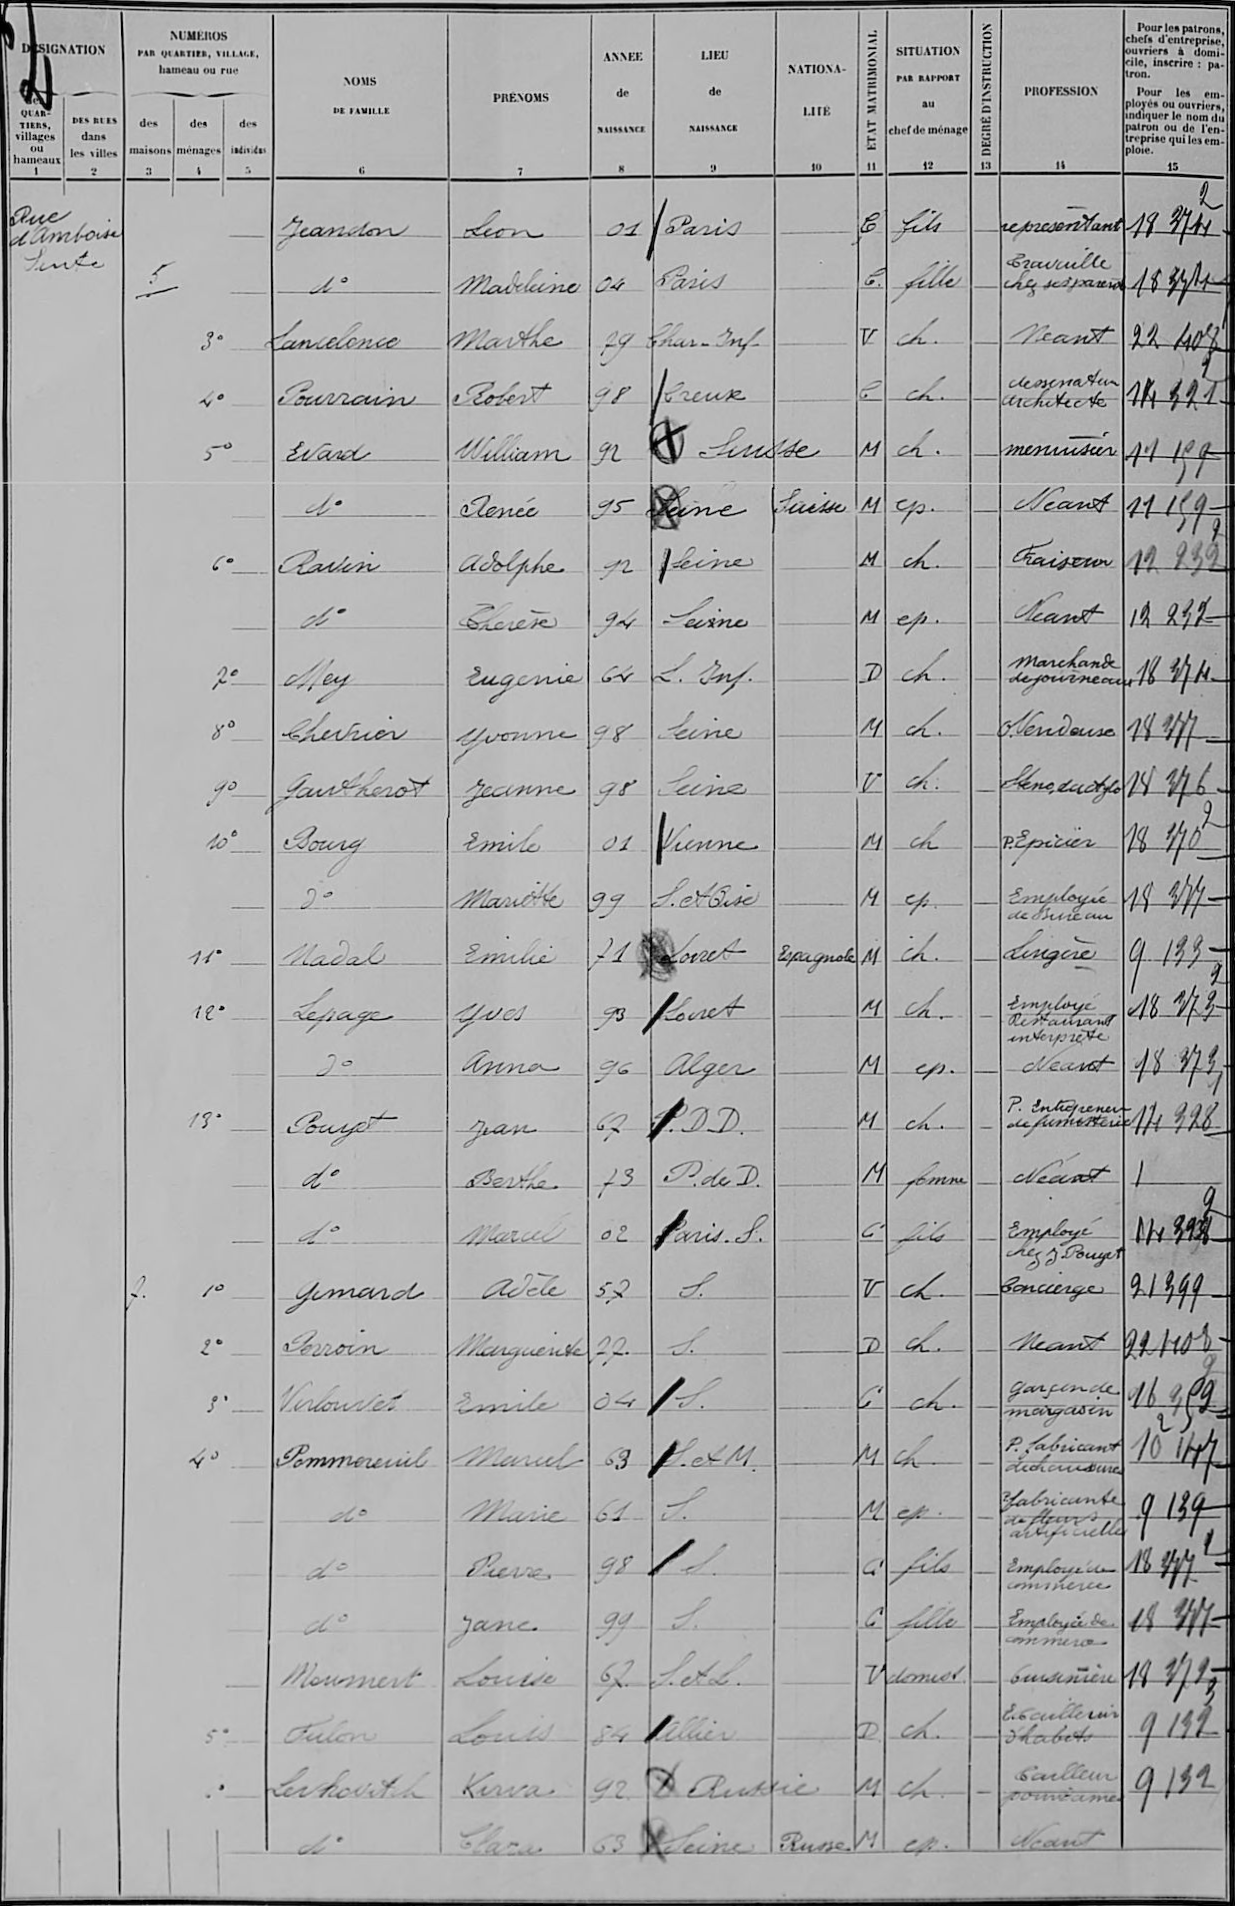Jeandon/Leon/01/Paris/¤/C/fils/¤/représentant/18374
d°/Madeleine/04/Paris/¤/C /fille/¤/chez ses parents?Travaille/¤/18374
Lancelonce/Marthe/79/Char Inf /¤/V/ch/¤/Neant/22408
Pourrain/Robert/98/Creuse/¤/C/ch/¤/architecte?dessinateur/14321
Evard/William/92/Suisse/¤/M/ch /¤/menuisier/11159
d°/Renée/95/Seine/Suisse/M/ep /¤/Neant/11159
Ravin/Adolphe/92/Seine/¤/M/ch/¤/Fraiseur/12239
d°/Thérèse/94/Seine/¤/M/ep /¤/Neant/12232
Mey/Eugenie/64/L Inf /¤/D/ch /¤/de journaux?Marchande/18374
Chevrier/Yvonne/98/Seine/¤/M/ch /¤/o Vendeuse/18377
Gautherot/Jeanne/98/Seine/¤/V/ch /¤/Sténo dactylo/18376
Bourg/Emile/01/Vienne/¤/M/ch/¤/P Epicier/18370
d°/Mariette/99/S et Oise/¤/M/ep/¤/Employée !de Bureau/18377
Nadal/Emilie/71/Loiret/Espagnole/M/ch /¤/Lingère/9133
Lepage/Yves/93/Loiret/¤/M/ch /¤/Employé!restaurant!Interprète/18373
d°/Anna/96/Alger/¤/M/ep /¤/Neant/18373
Pouyet/Jean/67/P D D /¤/M/ch /¤/de fumisterie?P Entrepreneur/14328
d°/Berthe/73/P de D /¤/M/femme/¤/Néant/¤
d°/Marcel/02/Paris S /¤/C/fils/¤/Employé! chez J Pouget/14398
Gemard/Adèle/57/S /¤/V/ch/¤/Concierge/21399
Perroin/Marguerite/77/S /¤/D/ch /¤/Neant/22408
Verlouvet/Emile/04/S /¤/C/ch /¤/magasin?garçon de/16459
Pommereuil/Marcel/63/S et M /¤/M/ch/¤/de chaussures?P fabricant/10147
d°/Marie/61/S /¤/M/ep /¤/de fleurs?fabricante!artificielles/9139
d°/Pierre/98/S /¤/C/fils/¤/Employé de! commerce/18377
d°/Jane/99/S /¤/C/fille/¤/Employée de! commerce/18377
Moumert/Louise/67/S et L /¤/V/domes/¤/cuisinière/18372
Fulon/Louis/84/Allier/¤/D/ch/¤/d'habits?E tailleur/9132
Levhovitch/Kirva/92/Russie/¤/M/ch /¤/pour dames?Tailleur/9132
d°/Clara/63/Seine/Russe/M/ep/¤/Néant/¤
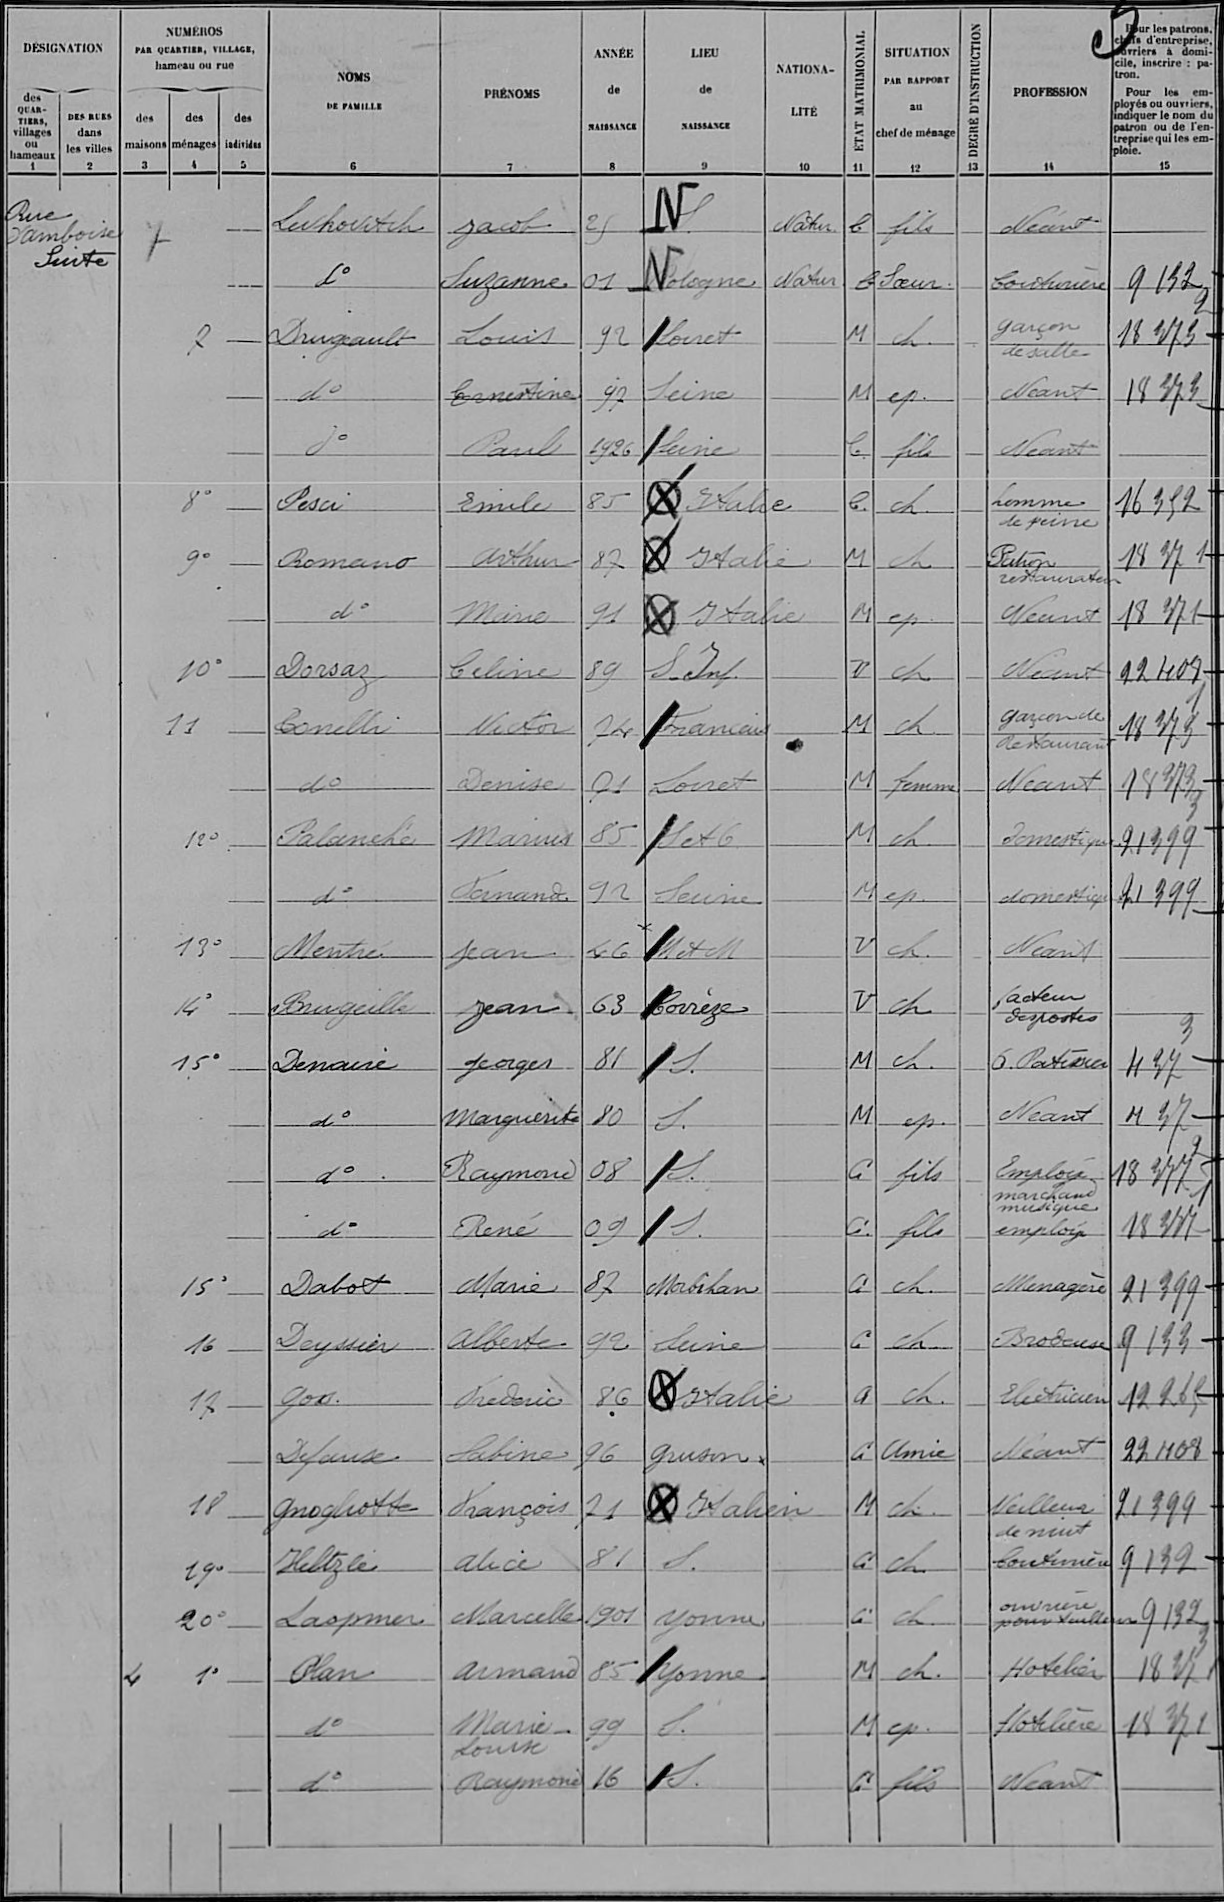Levhovitch/jacob/25/S /Natur/C/fils/¤/Néant/¤
d°/Suzanne/01/Pologne/Natur/C/Soeur/¤/Couturière/9132
Drugeault/Louis/92/Loiret/¤/M/ch /¤/garçon! de salle/18473
d°/Ernestine/97/Seine/¤/M/ep /¤/Neant/18473
d°/Paul/1926/Seine/¤/C/fils/¤/Neant/¤
Pesci/Emile/85/Italie/¤/C/ch /¤/homme! de peine/16352
Romano/Arthur/87/Italie/¤/M/ch/¤/Patron! restaurateur/18371
d°/Marie/91/Italie/¤/M/ep/¤/Neant/18371
Dorsaz/Celine/89/S Inf /¤/V/ch/¤/Neant/22409
Conelli/Victor/74/Français/¤/M/ch/¤/garçon! de restaurant/18373
d°/Denise/71/Loiret/¤/M/femme/¤/Neant/18373
Palanché/Marius/85/S et O/¤/M/ch /¤/domestique/21399
d°/Fernande/92/Seine/¤/M/ep/¤/domestique/21399
Mentré/jean/46/M et M/¤/V/ch /¤/Neant/¤
Brugeille/jean/63/Corrèze/¤/V/ch/¤/facteur! des postes/¤
Denaire/georges/81/S /¤/M/ch /¤/O Patissier/437
d°/Marguerite/80/S /¤/M/ep /¤/Neant/437
d°/Raymond/08/S /¤/C/fils/¤/Employé! marchand!musique/18377
d°/René/09/S /¤/C/fils/¤/employé/18377
Dabot/Marie/87/Morbihan/¤/C/ch /¤/Ménagère/21399
Deyssier/Alberte/92/Seine/¤/C/ch/¤/Brodeuse/9133
Goss/Frederic/86/Italie/¤/C/ch /¤/Electricien/12265
Defause/Sabine/96/Gruson/¤/C/Amie/¤/Neant/22408
Gnoghosse/François/71/Italien/¤/M/ch/¤/Veilleur! de nuit/21399
Heltzle/alice/81/S /¤/C/ch/¤/Couturière/9132
Laspmer/Marcelle/1901/Yonne/¤/C/ch /¤/ouvrière! pour veilleur/9132
Plan/Armand/85/Yonne/¤/M/ch /¤/Hotelier/18371
d°/Marie!Louise/99/S /¤/M/ep /¤/Hotelière/18371
d°/Raymond/16/S /¤/C/fils/¤/Neant/¤